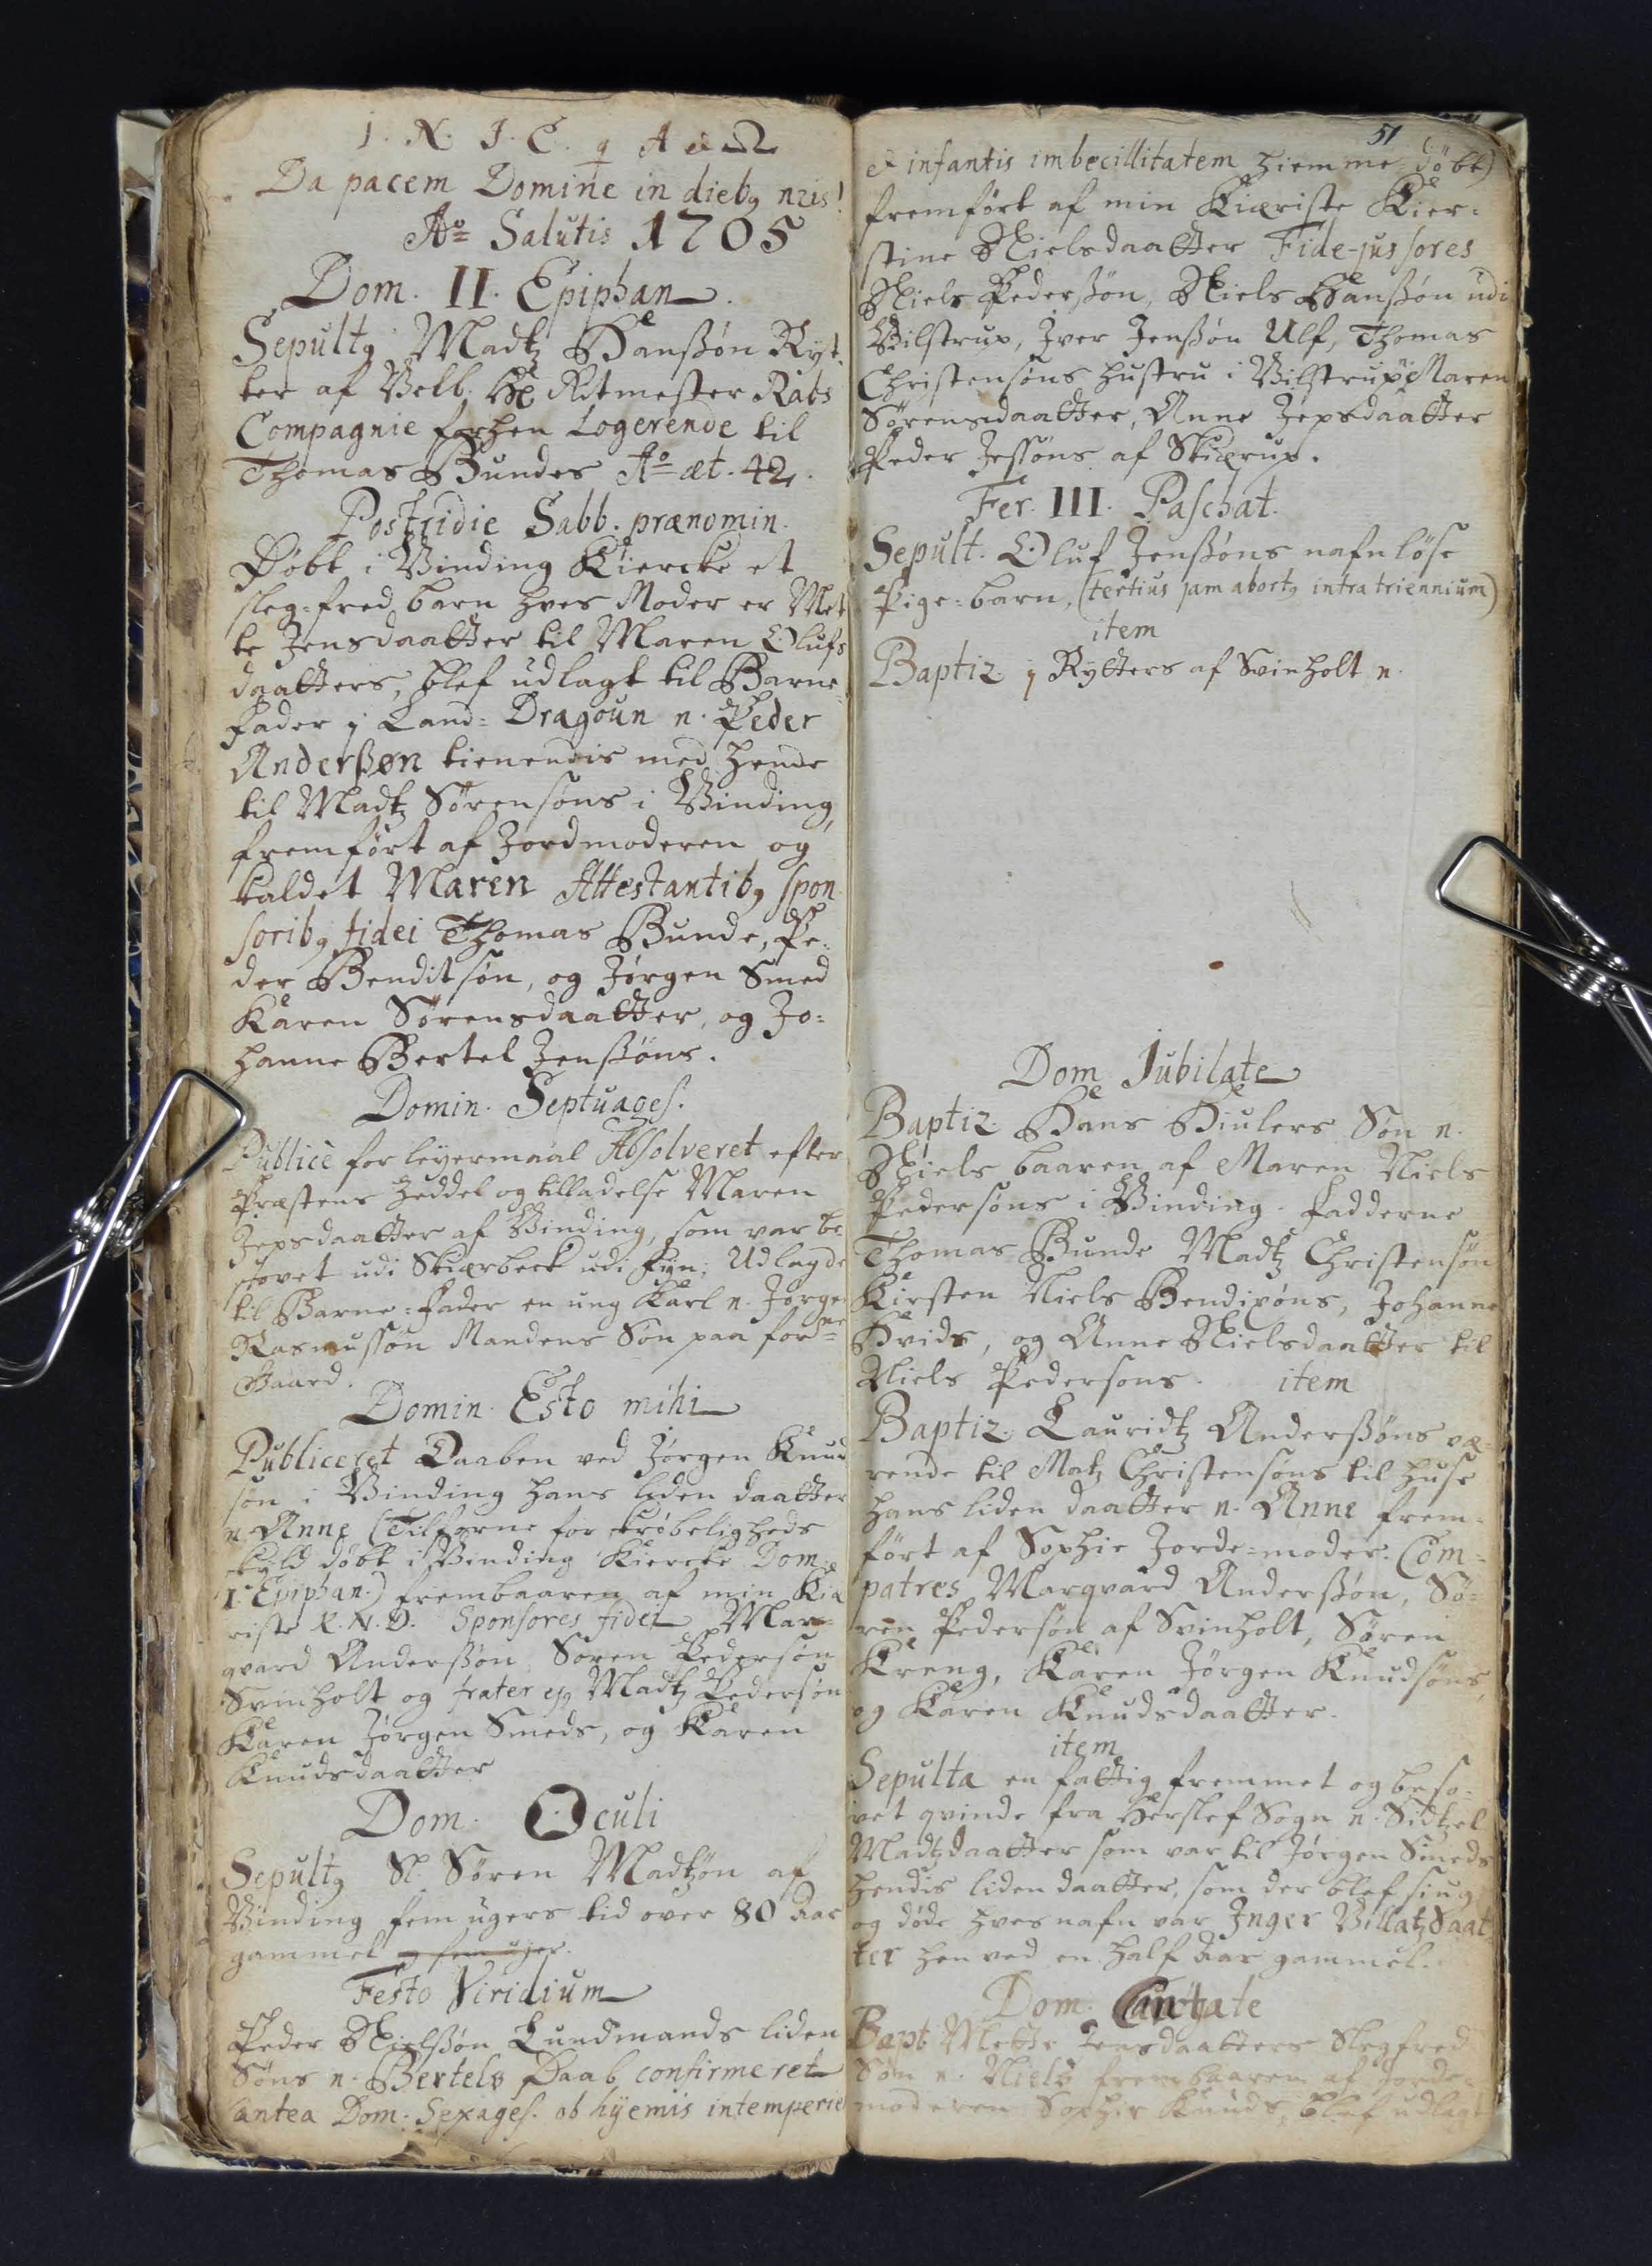I. N. J. C. q A et Ω
Da pacem Domine in dirbq nzis!
Ao Salutis 1705
Dom. II. Epiphan
Sepultq Madtz Hanssøn Ryt
ter af Vell, Hl Ritmester Rabs
Compagnie, forhen Logerende til 
Thomas Bundes Ao æt. 42
Postridie Sabb. prænomin.
Døbt i Vinding Kiercke et
sleg=fred barn hves Moder er Met
te Jensdaatter til Maren Olufs
daatters, blef udlagt til Barne
Fader 1 Land= Dragoun n. Peder
Anderssøn tienendis med hende
til Madtz Sørensøns i Vinding,
fremført af Jordemoderen og
kaldet Maren Attestantibq spon
soribq fidei Thomas Bunde, Pe¬
der Benditsøn, og Jørgen Smed
Karen Sørensdaatter, og Jo¬
hanne Bertel Jenssøns.
Domin Septuages.
Publice for leyermaal Absolveret efter
Præstens Zeddel og tilladelse Maren
Jepsdaatter af Vinding, som var be
sovet udi Skiærbeck udi Fyn; Udlage
til Barne = Fader en ung Karl n. Jørge 
Rasmussøn Mandens Søn paa forne
Gaard.
Domin Esto mihi
Publiceret Daaben ved Jørgen Knud
søn i Vinding hans liden daatter
n. Anne (tilforne for skrøbeligheds
skyld døbt i Vinding Kiercke Dom
I Epiphan.) frembaaren af min Kiæ
riste K.N.D. Sponsores fidei Mar¬
qvard Anderssøn, Søren Pedersøn
Svinholt, og frater## es## Madtz Pedersøn
Karen Jørgen Smeds, og Karen
Knudsdaatter
Dom. Oculi
Sepultq Sl. Søren Madtzøn af
Vinding fem ugers tid over 80 Aar
gammel og fem uger.
Festo Viridium
Peder Nielssøn Lundmands liden
Søns n. Bertels Daab confirmeret
(antea Dom. Sexages. ob hyemis intemperie 
51
et infantis imbecillitatem Hiemme=døbt)
fremført af min Kiæriste Kier¬
stine Nielsdaatter. Fide-jussores
Niels Pederssøn, Niels Hanssøn udi
Vilstrup, Iver Jenssøn Ulf, Thomas
n.
Christensøns hustru i Vilstrup Maren
Sørensdaatter, Anne Jepsdaatter
Peder Jessøn af Skiærup.
Fer. III Paschat.
Sepult. Oluf Jenssøns nafnløse
Pige=barn, (tertius jam abort intra trieanium)
item
Baptiz. 1 Rytters af Svinholt n. 
Dom. Jubilate
Baptiz. Hans Hiulers Søn n.
Niels baaren af Maren Niels
Pedersøns i Vinding. Fadderne
Thomas Bunde Madtz Christensøn
Kirsten Niels Bendixøns, Johanne
Hvids, og Anne Nielsdaatter til
Niels Pedersons. item
Baptiz. Lauridtz Anderssøns væ¬
rende til Matz Christensøns til huse
hans liden daatter n. Anne, frem¬
ført af Sophie Jorde=moder. Com¬
patres Marqvard Anderssøn, Sø¬
ren Pedersøn af Svinholt, Søren
Kreng, Karen Jørgen Knudsøns
og Karen Knudsdaatter
item
Sepulta en fattig fremmet og beso¬
vet qvinde fra Herslef Sogn n. Sidtzel 
Madtzdaatter som var til Jørgen Smeds
hendis liden daatter, som der blef siug
og døde hves nafn var Inger Villatzdaat
ter hen ved en half Aar gammel.
Dom. Cantate
Bapt Mette Jensdaatters Slegfred
Søn n. Niels, frembaaren af Jorde¬
moderen Sophie Knuds, blef udlagt